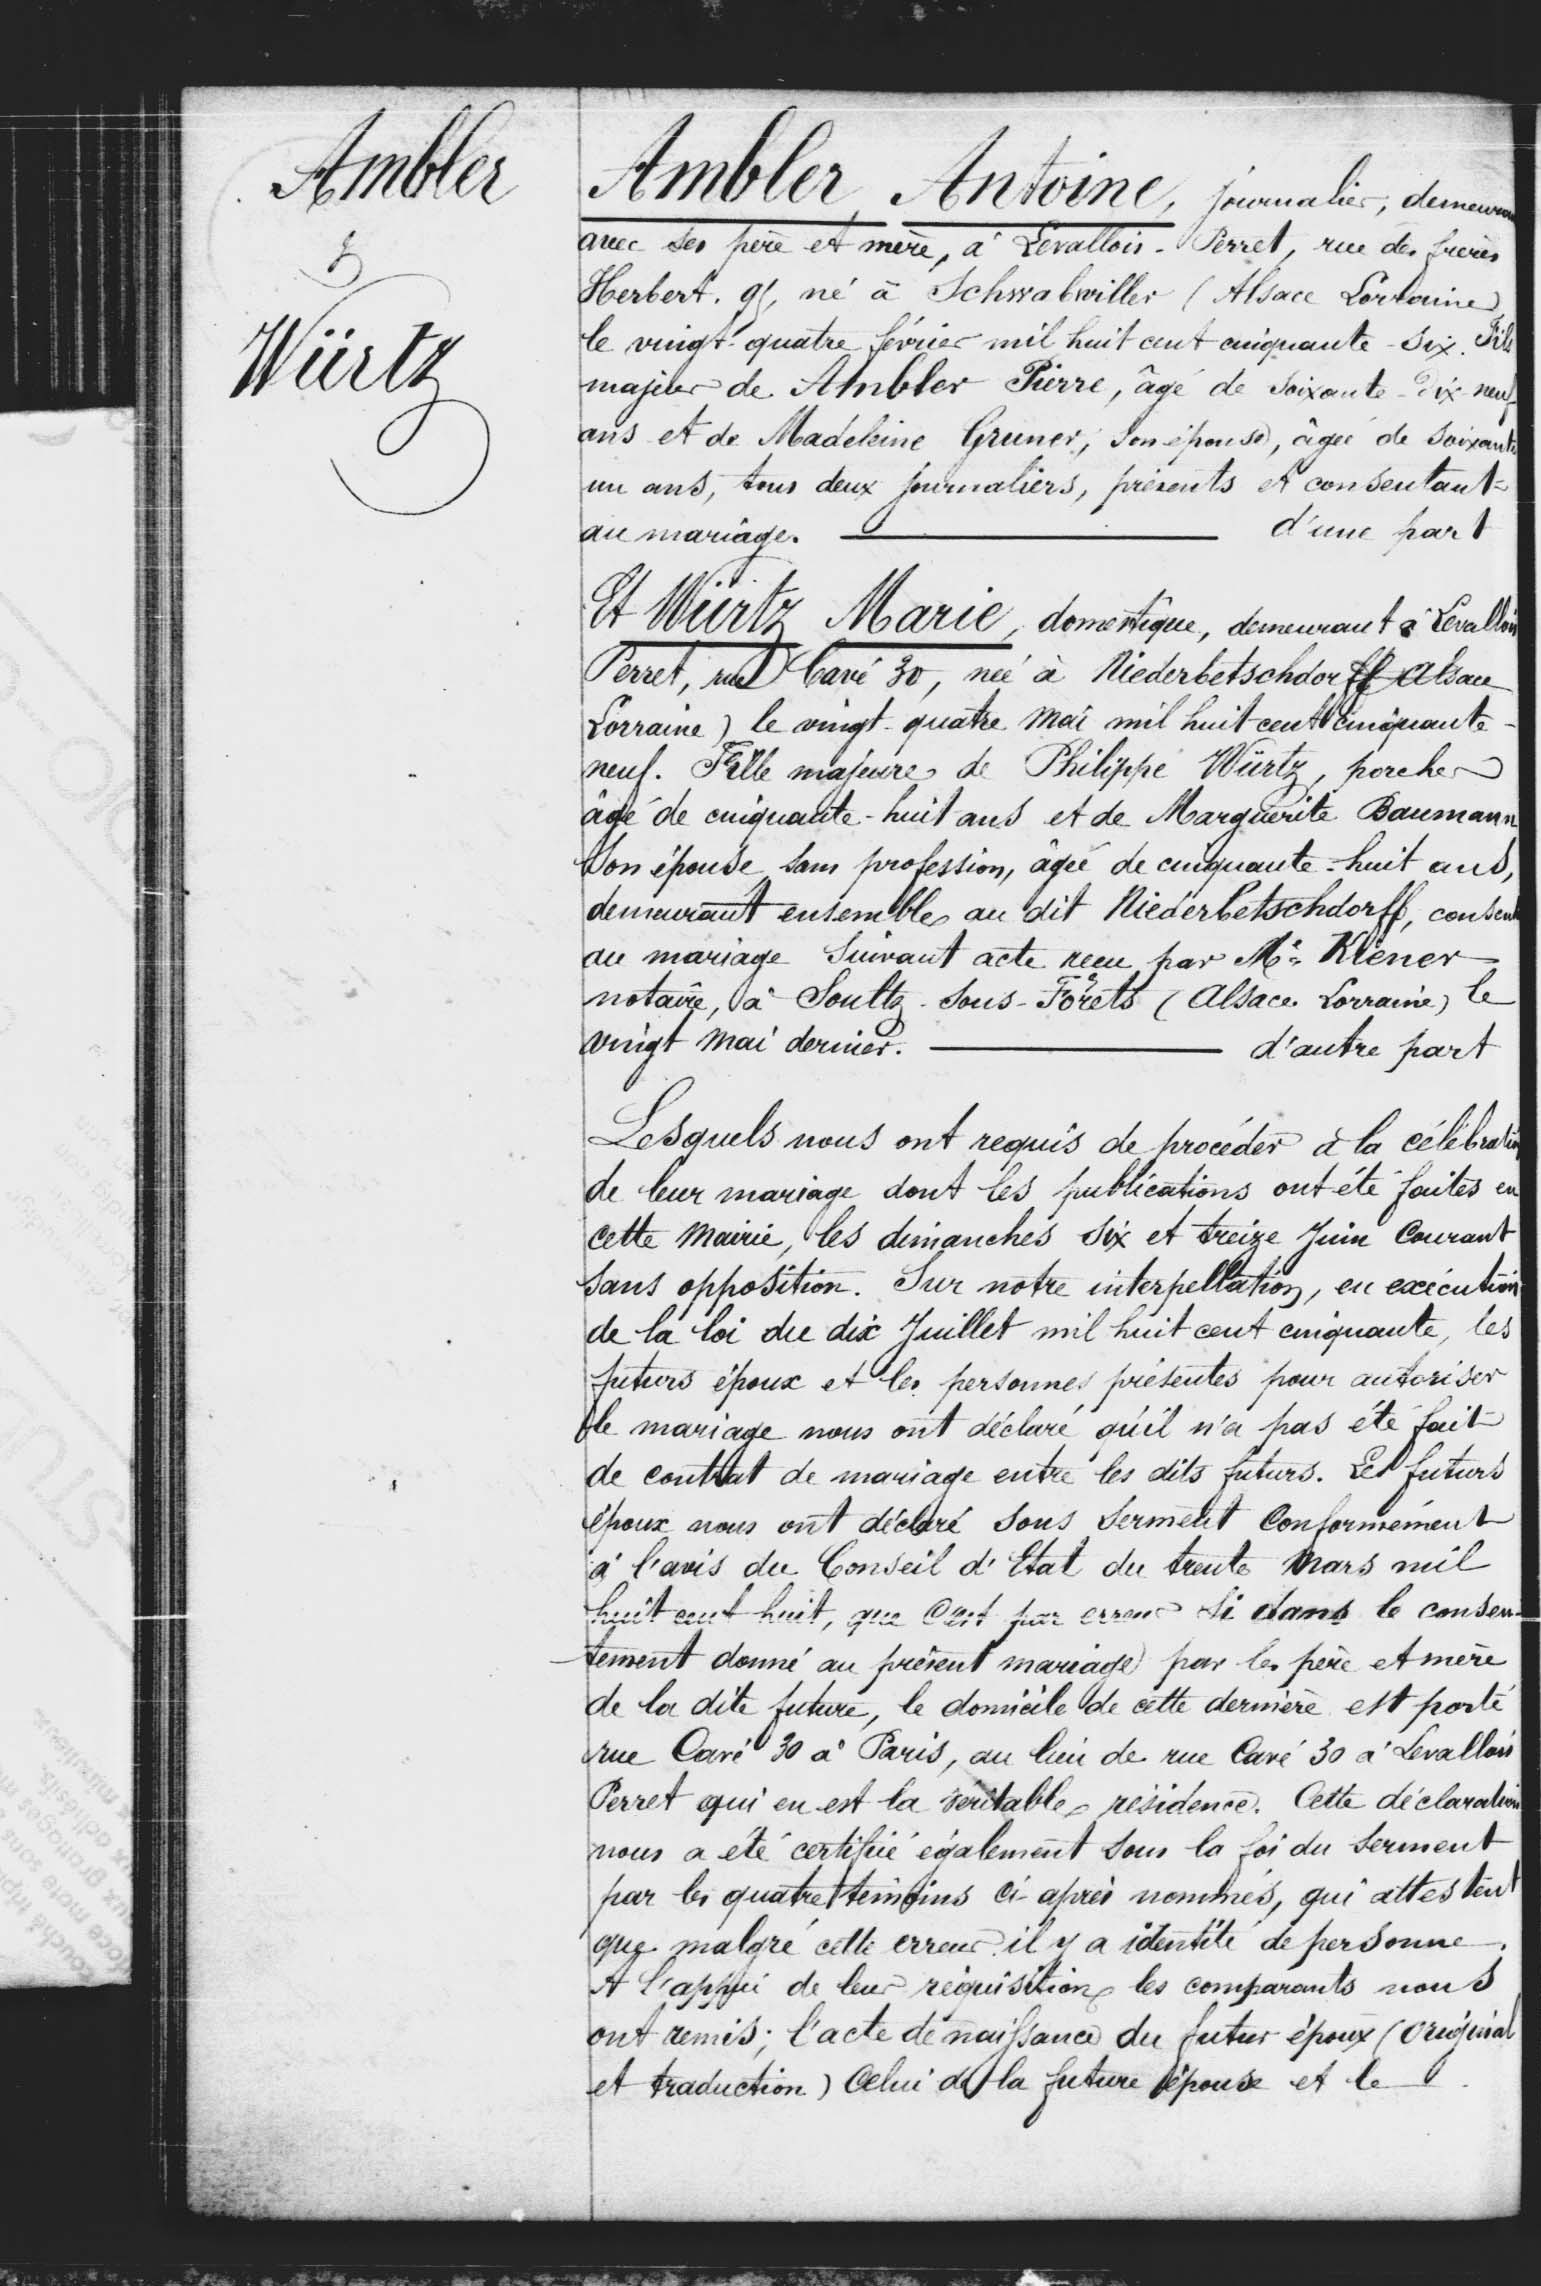Ambler
&
Würtz
Ambler, Antoine, journalier demeurant
avec ses père et mère à Levallois-Perret, rue des frères
Herbert, 95, né à Schssabwiller (Alsace Lorraine)
le vingt-quatre février mil huit cent cinquante-six. Fils
majeur de Ambler Pierre, âgé de soixante-dix-neuf
ans et de Madeleine Gruner, son époue, âgée de soixante
un ans, tous deux journaliers, présents et consentants
au mariage. -d'une part
Et Würtz Marie, domestique, demeurant à Levallois
Perret, rue Cavé 30, née à Niederbetschdor (Alsace
Lorraine) le vingt-quatre mai mil huit cent cinquante-
neuf. Fille majeure de Philippe Würtz, porcher
âgé de cinquante-huit ans et de Marguerite Bausmann
son épouse sans profession, âgée de cinquante-huit ans,
demeurant ensembles au dit Niederbetschdorff, consent
au mariage suivante acte reçu par Me Kléner
notaire, à Soultz-sous-Forêts (Alsace Lorraine) le
vingt mai dernier. -d'autre part
Lesquels nous ont requis de procéder à la célébration
de leur mariage dont les publications ont été faites en
cette mairie, les dimanches six et treize juin courant
sans opposition. Sur notre interpellation, en exécutiton
de la loi du dix juillet mil huit cent cinquante, les
futurs époux et les personnes présentes pour autoriser
le mariage nous ont déclaré qu'il n'a pas été fait
de contrat de mariage entre les dits futurs. Les futurs
époux nous ont déclaré sous serment conformément
à l'avis du Conseil d'Etat du trente mars mil
huit cent huit, que ceux par erreur si dans le consen-
tement donné au présent mariage par les père et mère
de la dite future, le domicile de cette dernière est porté
rue Caré 30 à Paris, au lieu de rue Caré 30 à Levallois
Perret qui en est la véritable résidence. Cette déclaration
nous a été certifié également sous la foi du serment
par les quatre témoins ci après nommés, qui attesent
que malgré cette erreur il y a identité de personne.
A l'appui de leur réquisition les comparants nous
ont remis l'acte de naissance du futur époux (original
et traduction) celui de la future épouse et le
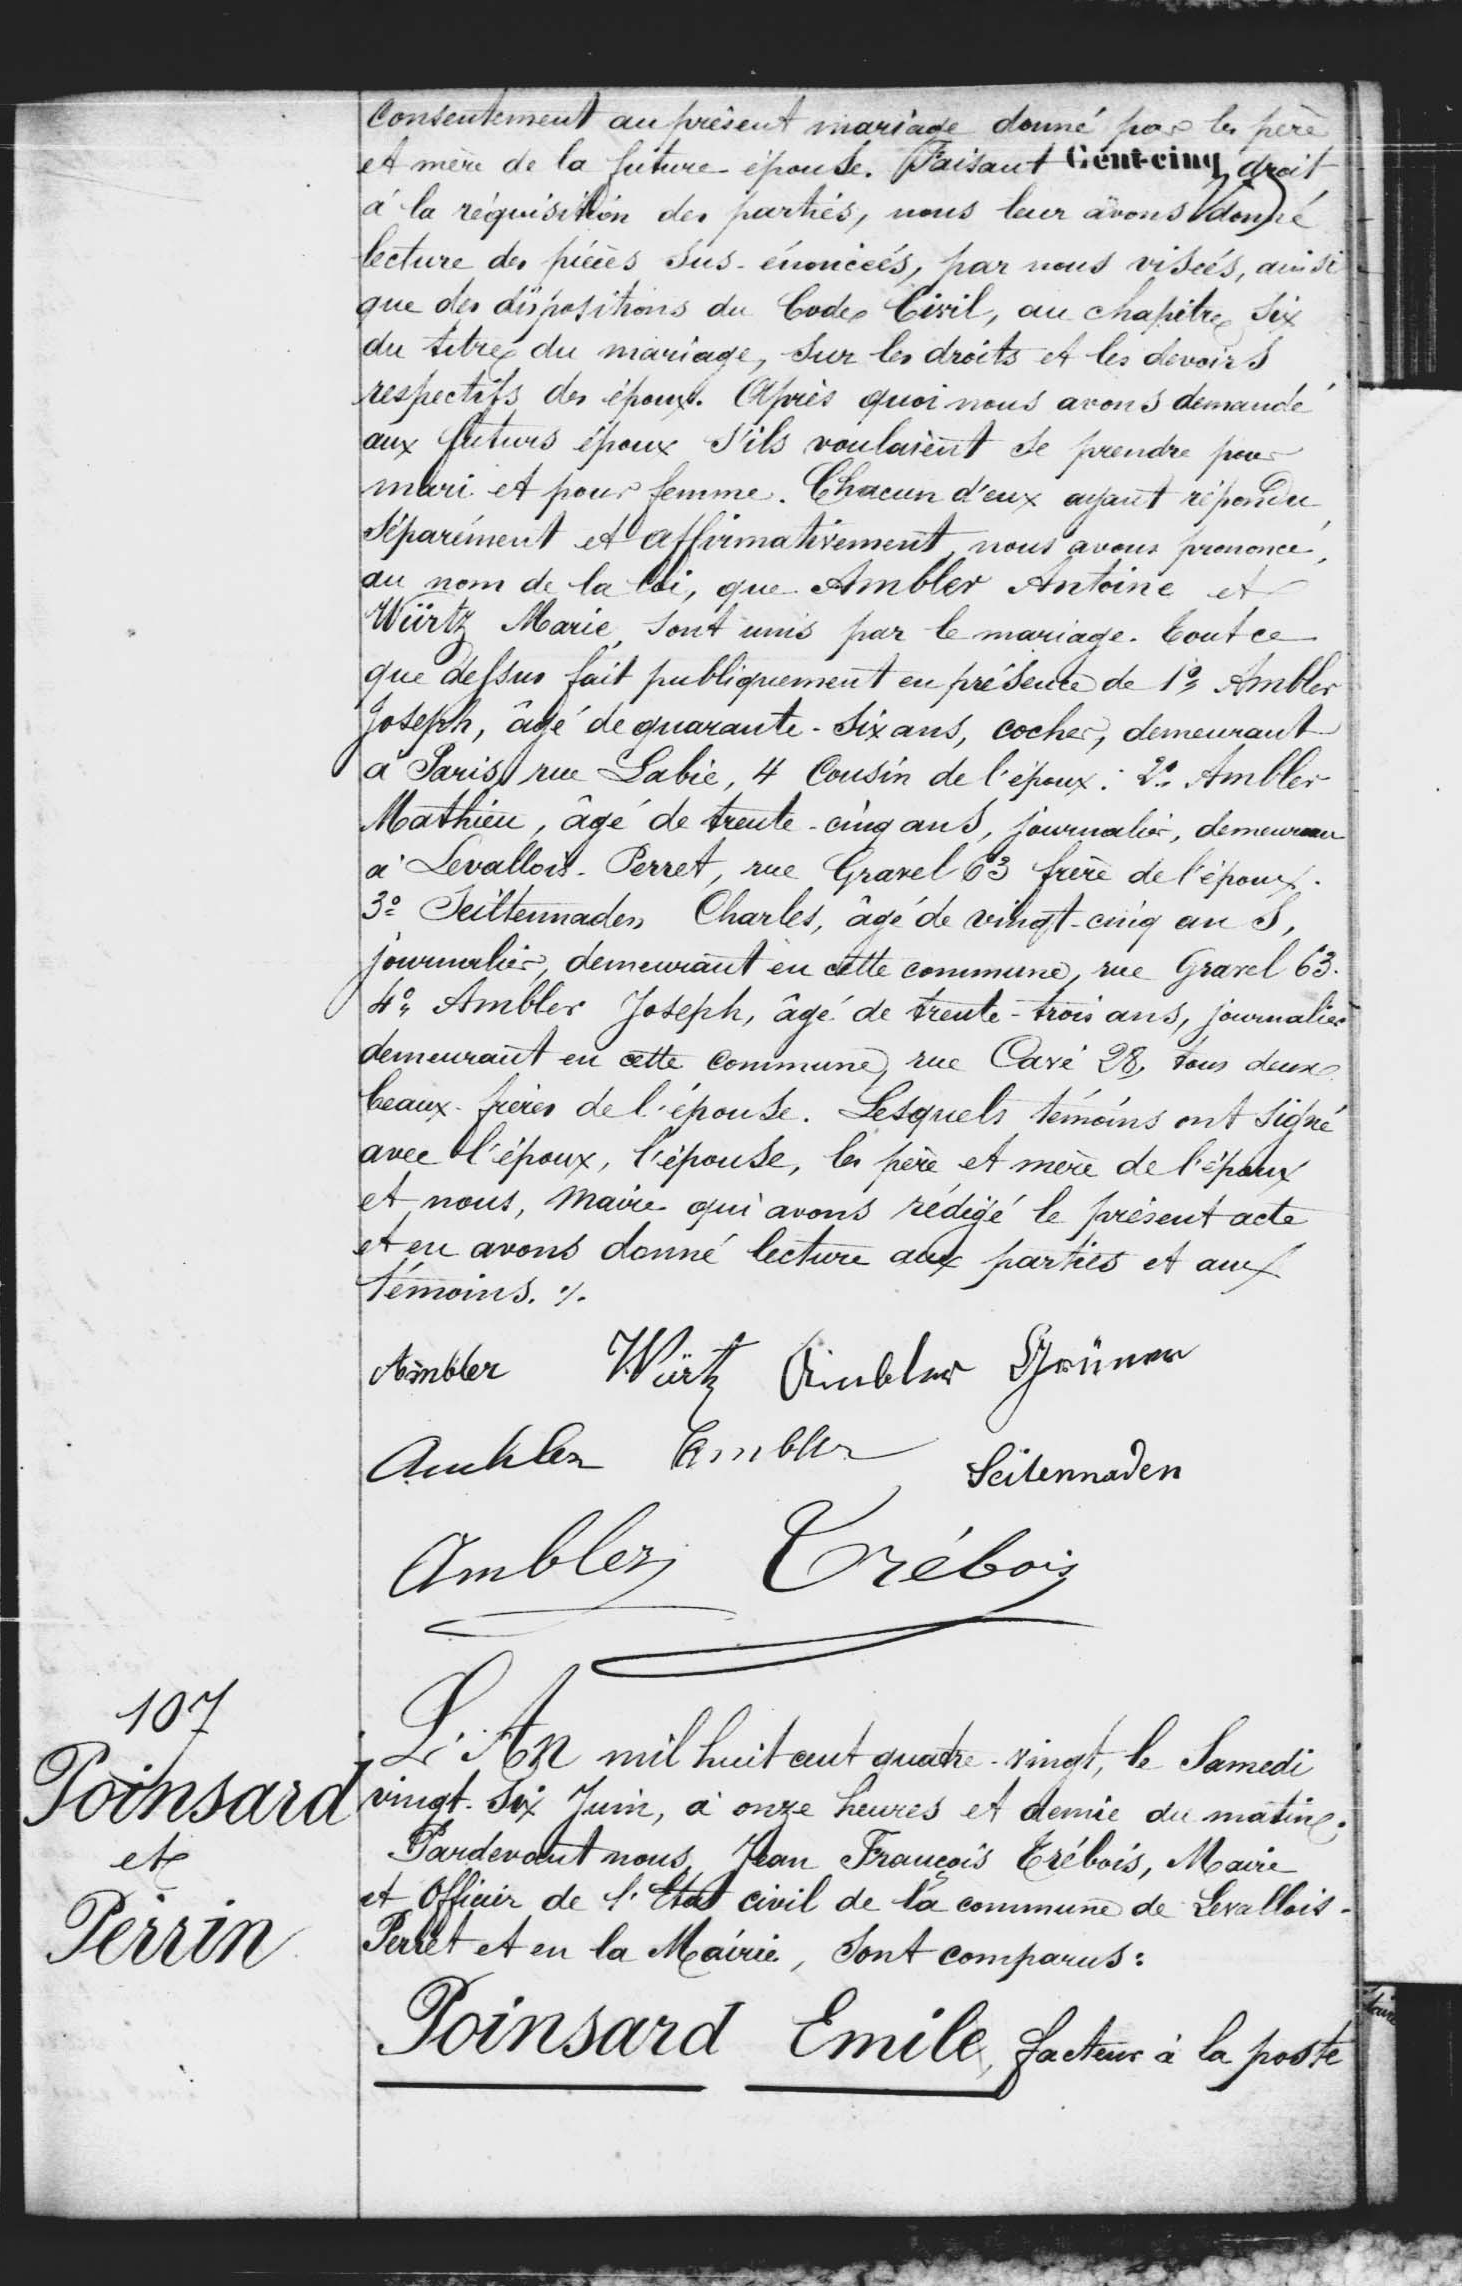consentement au présent mariage donné par les père
et mère de la future épouse faisant cent cinq droit
à la réquisition des parties, nous leur avons donné
lecture des pièces sous-énoncées, par nous visées, ainsi
que des dispositions du Code Civil, au chapitre six
du titre du mariage, sur les droits et les devoirs
respectifs des époux. Après quoi nous avons demandé
aux futurs époux s'ils voulaient se prendre pour
mari et pour femme. Chacun d'eux ayant répondu
séparément et d'affirmativement nous avons prononcé,
au nom de la loi, que Amblert Antoine et
Würtz Marie sont unis par le mariage. tout ce
que dessus fait publiquement en présence de 1er Amble
Joseph, âgé de quarante-six ans, cocher, demeurant
à Paris rue Sabie, 4 Cousin de l'époux, 2e Ambler
Mathieu, âgé de trente-cinq ans, journalier, demeurant
à Levallois-Perret, rue Gravel 63 frère de l'époux.
3e Seilternaden Charles, âgé de vingt-cinq ans,
journalier, demeurant en cette commune, rue Gravel 63.
4e Ambler Joseph, âgé de trente-trois ans, journalier
demeurant en cette commune, rue Caré 28, tous deux
beaux-frères de l'épouse. Lesquels témoins ont signé
avec l'époux, l'épouse, les père et mère de l'époux
et nous, maire qui avons rédigé le présent acte
et en avons donné lecture aux parties et aux
témoins. ./.
107
Poinsard
et
Perrin
L'An mil huit cent quatre-vingt le samedi
vingt-six juin, à onze heures et demie du matin.
Pardevant ous Jean François Trébois, Maire
et Officier de l'Etat civil de la commune de Levallois-
Perret et en la Mairie, sont comparus:
Poinsard Emile, facteur à la poste